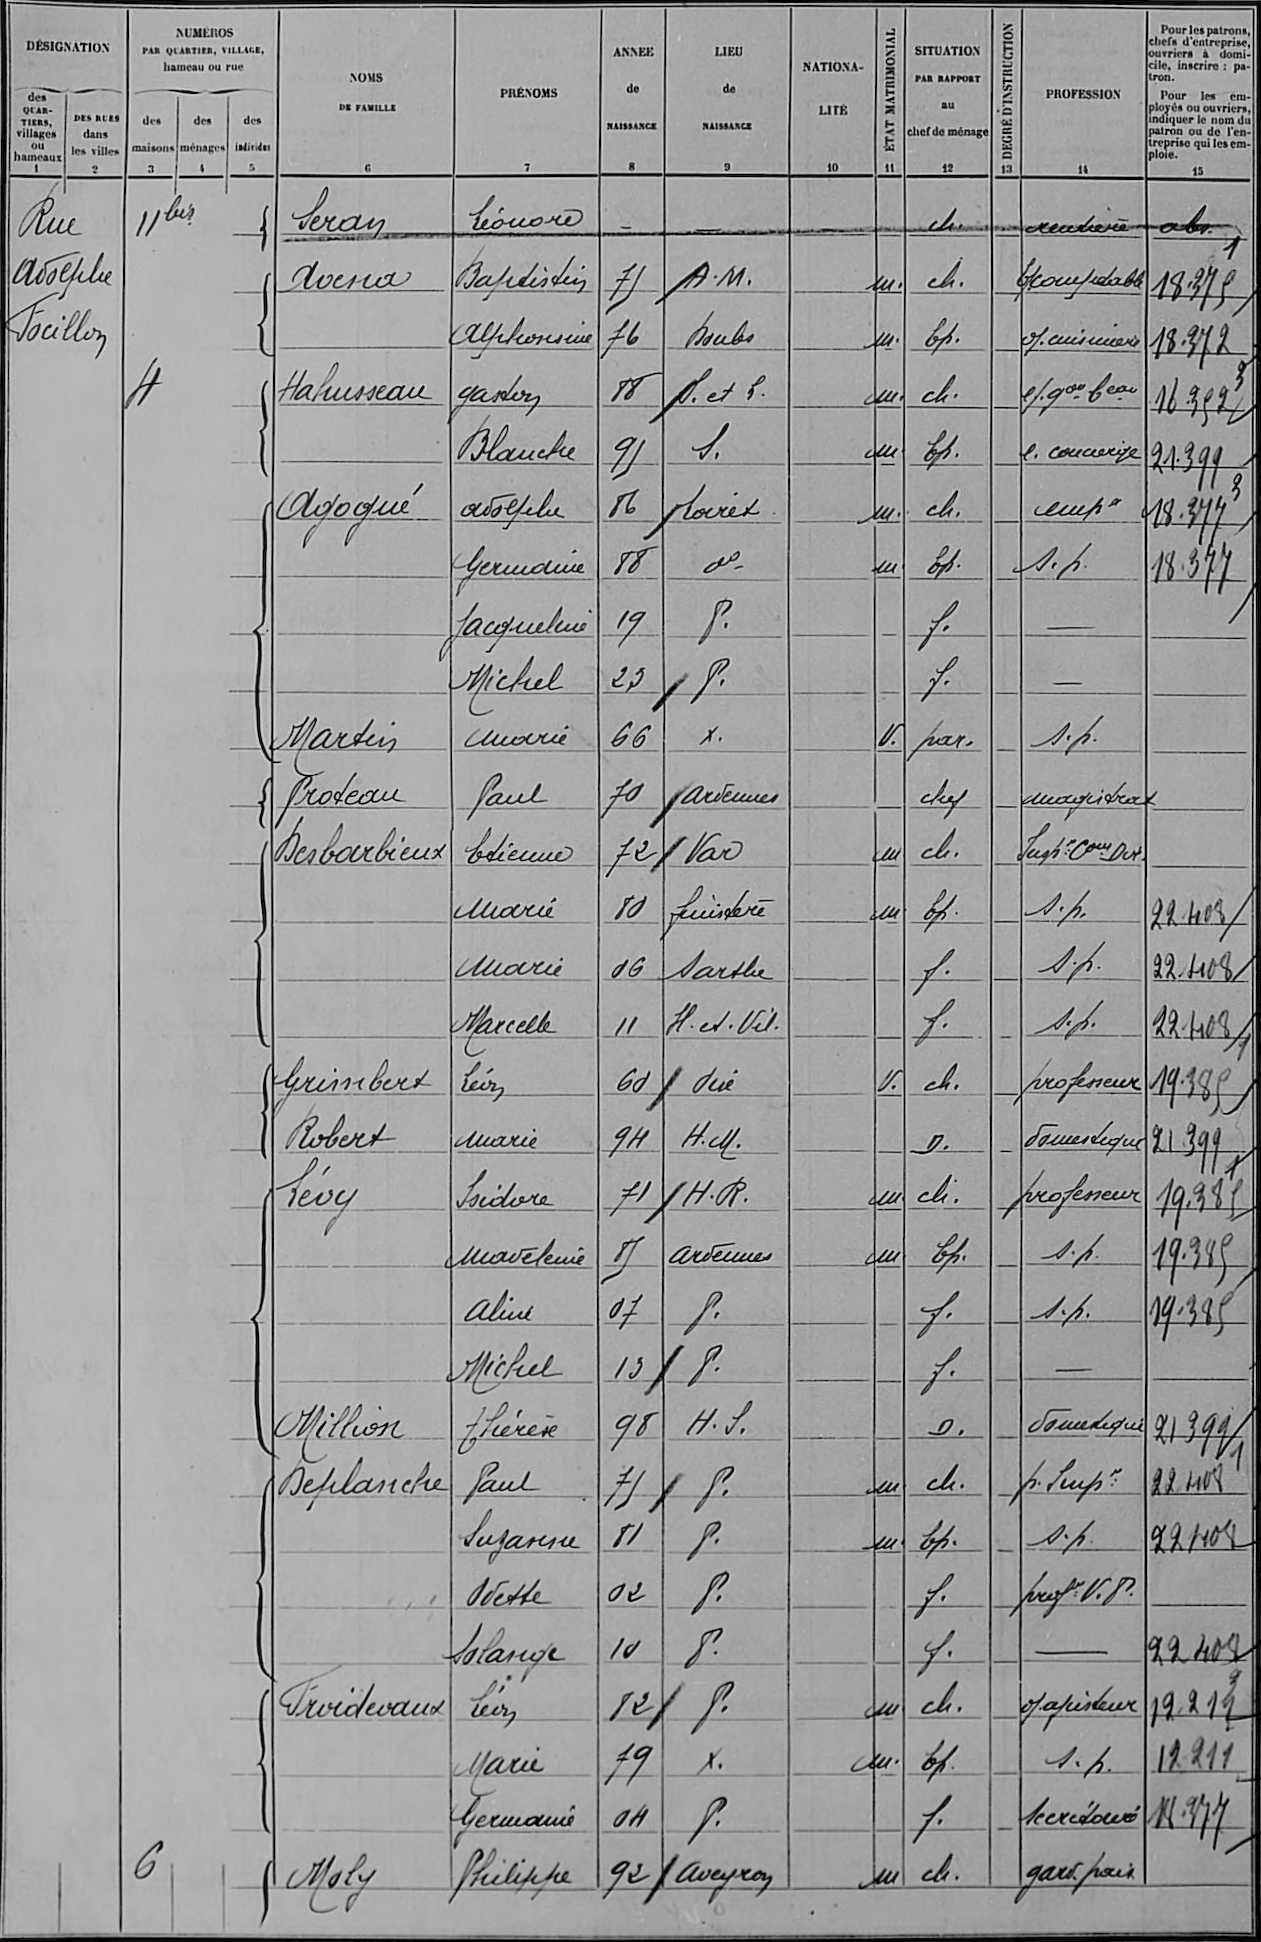Seran/Léonore/¤/¤/¤/¤/ch /¤/rentière/abs
Avena/Bafidisdin/71/A M /¤/m /ch /¤/E Comptable/18373
¤/Alphonsine/76/Doubs/¤/m /Ep /¤/o cuisinière/18372
Hahusseau/Gaston/88/S et h /¤/m /ch /¤/e gon Beau/16352
¤/Blanche/91/S /¤/m /Ep /¤/e concierge/21399
Agogué/adolphe/86/Loiret/¤/m /ch /¤/emp/18377
¤/Germaine/88/d°/¤/m /Ep /¤/s p /18377
¤/Jacqueline/19/P /¤/¤/f /¤/¤/¤
¤/Michel/23/P /¤/¤/f /¤/¤/¤
Martin/Andrée/66/X /¤/V /par /¤/s p /¤
Prodeau/Paul/70/ardennes/¤/¤/chef/¤/magistrat/¤
Desbarbieux/Etienne/72/Var/¤/m/ch /¤/Inspr Com Dir/¤
¤/Marie/80/finistère/¤/m/Ep /¤/s p /22408
¤/Marie/06/Sarthe/¤/¤/f /¤/s p /22408
¤/marcelle/11/I et Vil/¤/¤/f /¤/s p /22408
Grimberd/Léon/60/Oise/¤/V /ch /¤/professeur/19385
Robert/Marie/94/H M /¤/¤/D /¤/domestique/21399
Lévy/Isidore/71/H R /¤/m/ch /¤/professeur/19385
¤/Madeleine/87/Ardennes/¤/m /Ep /¤/s p /19389
¤/aline/07/P /¤/¤/f /¤/s p /19385
¤/Michel/13/P /¤/¤/f /¤/¤/¤
Million/Thérèse/98/H S /¤/¤/D /¤/domestique/21399
Deplanche/Paul/71/P /¤/m/ch /¤/p Impr/22408
¤/Suzanne/81/P /¤/m /Ep /¤/s p /22408
¤/Odette/02/P /¤/¤/f /¤/prof V d/¤
¤/Solange/10/P /¤/¤/f /¤/¤/22408
Froideveaux/Léon/82/P /¤/m/ch /¤/o ajusteur/12299
¤/Marie/79/X /¤/m /Ep /¤/s p /12211
¤/Germaine/04/P /¤/¤/f /¤/secrétaire/18377
Moly/Philippe/92/Aveyron/¤/m/ch /¤/gard paix/¤
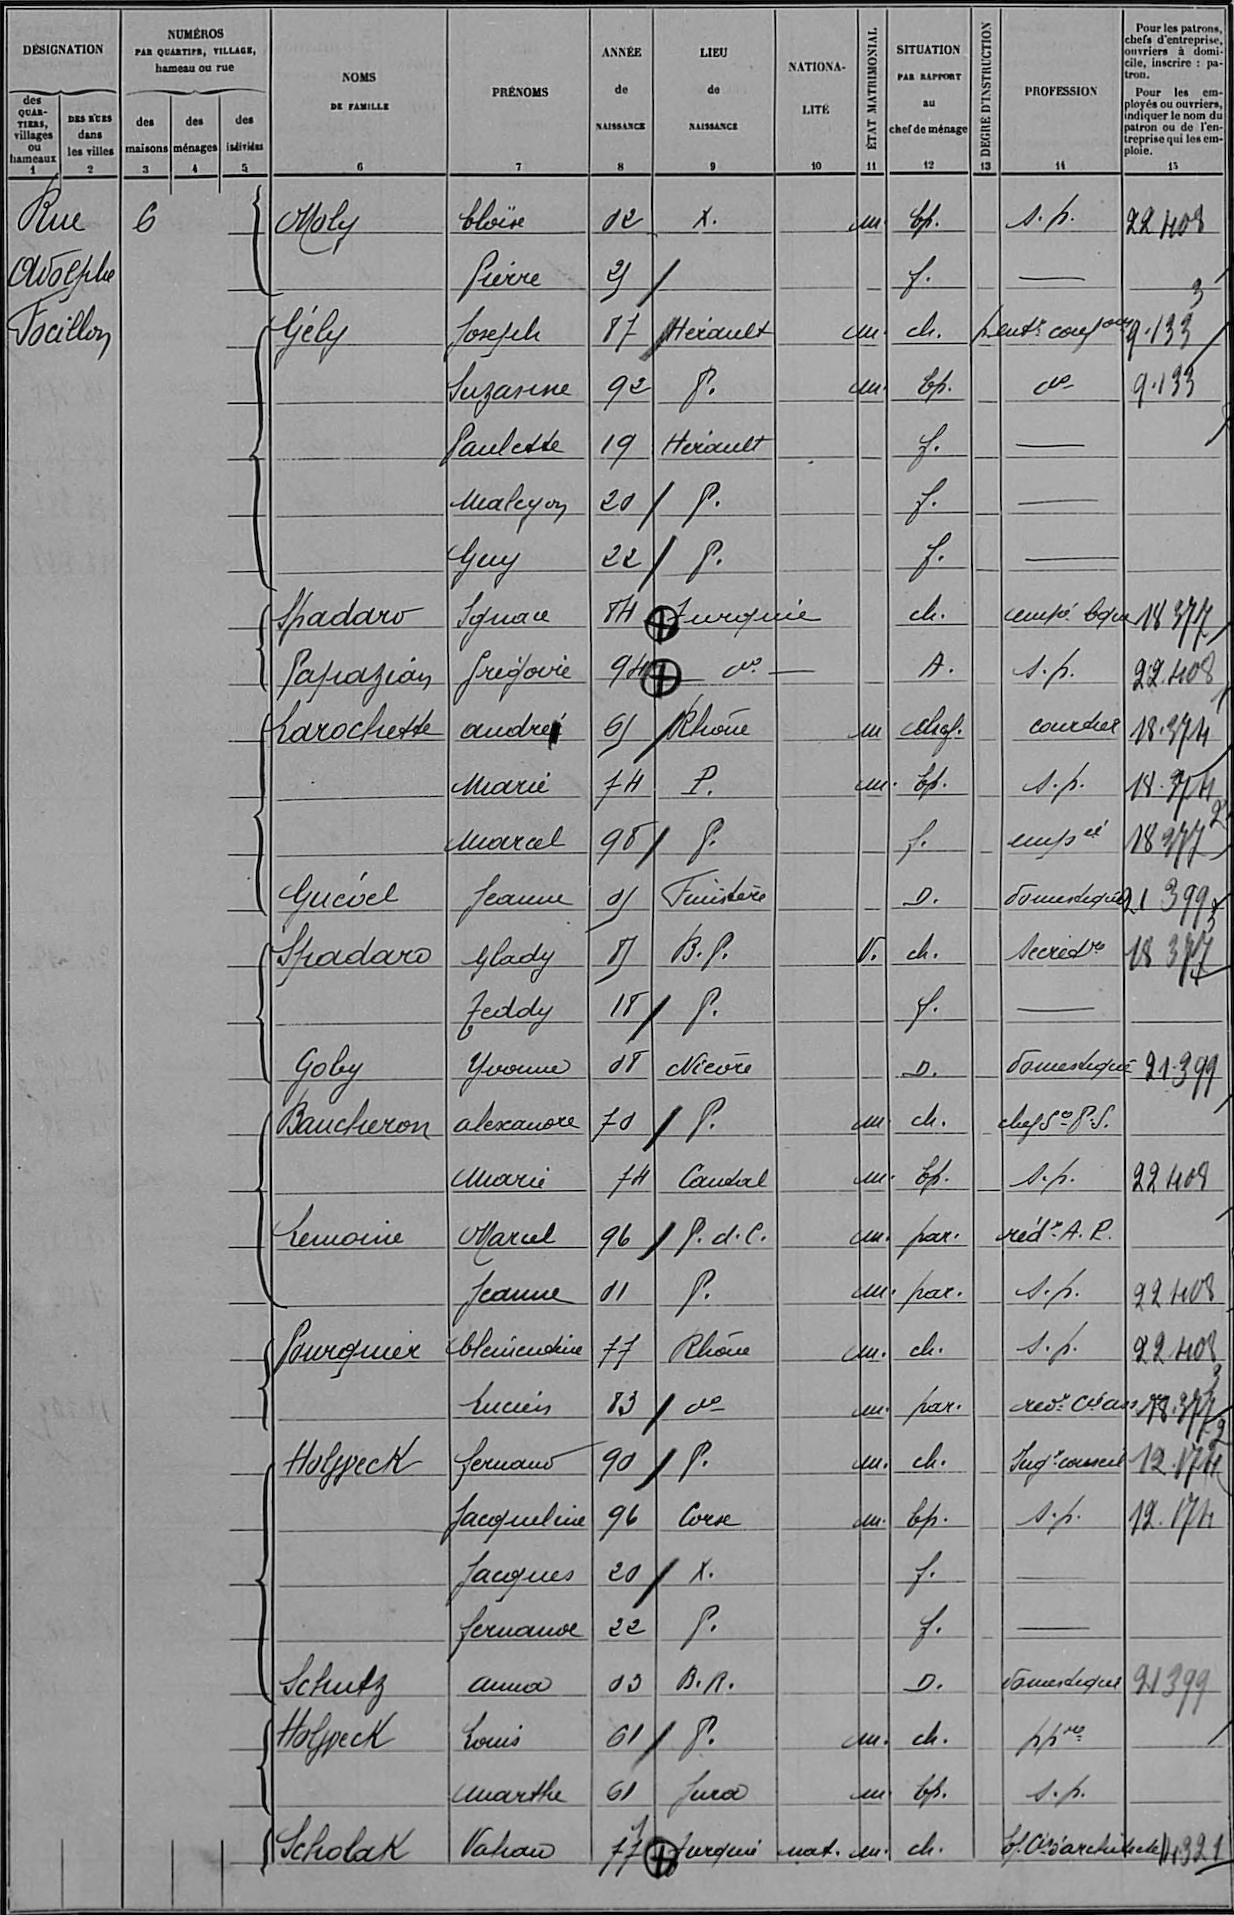Moly/Eloïse/02/X /¤/m/Ep /¤/s p /22408
¤/Pierre/21/¤/¤/¤/f /¤/¤/¤
Gély/Joseph/87/Hérault/¤/m /ch /¤/p enr confon/9133
¤/Suzanne/92/P /¤/m /Ep /¤/d°/9133
¤/Paulette/19/Hérault/¤/¤/f /¤/¤/¤
¤/Maleyon/20/P /¤/¤/f /¤/¤/¤
¤/Guy/22/P /¤/¤/f /¤/¤/¤
Spadaro/Ignace/84/Turquie/¤/¤/ch /¤/emp bque/18377
Papazian/Grégoire/94/d°/¤/¤/A /¤/s p /22408
Larochette/André/61/Rhone/¤/m/chef /¤/courtier/18374
¤/Marie/74/P /¤/m/Ep /¤/s p /18374
¤/Marcel/98/P /¤/¤/f /¤/emp/18377
Guével/Jeanne/01/Finistère/¤/¤/D /¤/domestique/21399
Spadaro/Glady/81/B P /¤/V /ch /¤/Secretre/18377
¤/Teddy/18/P /¤/¤/f /¤/¤/¤
Goby/Yvonne/08/Nièvre/¤/¤/D /¤/domestique/21399
Baucheron/alexandre/70/P /¤/m /ch /¤/chef Sce P S /¤
¤/Marie/74/Cantal/¤/m /Ep /¤/s p /22408
Lemoine/Marcel/96/P d C /¤/m /par /¤/réd A P /¤
¤/Jeanne/01/P /¤/m /par /¤/s p /22408
Pourquier/blémendine/77/Rhône/¤/m /ch /¤/s p /22408
¤/Lucien/83/d°/¤/m /par /¤/recr cre ass/18377
Holyveck/fernand/90/P /¤/m /ch /¤/Jug conseil/12174
¤/Jacqueline/96/Corse/¤/m /Ep /¤/s p /12174
¤/Jacques/20/X /¤/¤/f /¤/¤/¤
¤/féruauve/22/P /¤/¤/f /¤/¤/¤
Schutz/anna/03/B R /¤/¤/D /¤/domestique/21399
Holweck/Louis/61/P /¤/m /ch /¤/p pre/¤
¤/Marthe/61/Jura/¤/m /Ep /¤/s p /¤
Scholak/Vahan/77/turquie/nat /m /ch /¤/Ep Cis d'architecte/14321